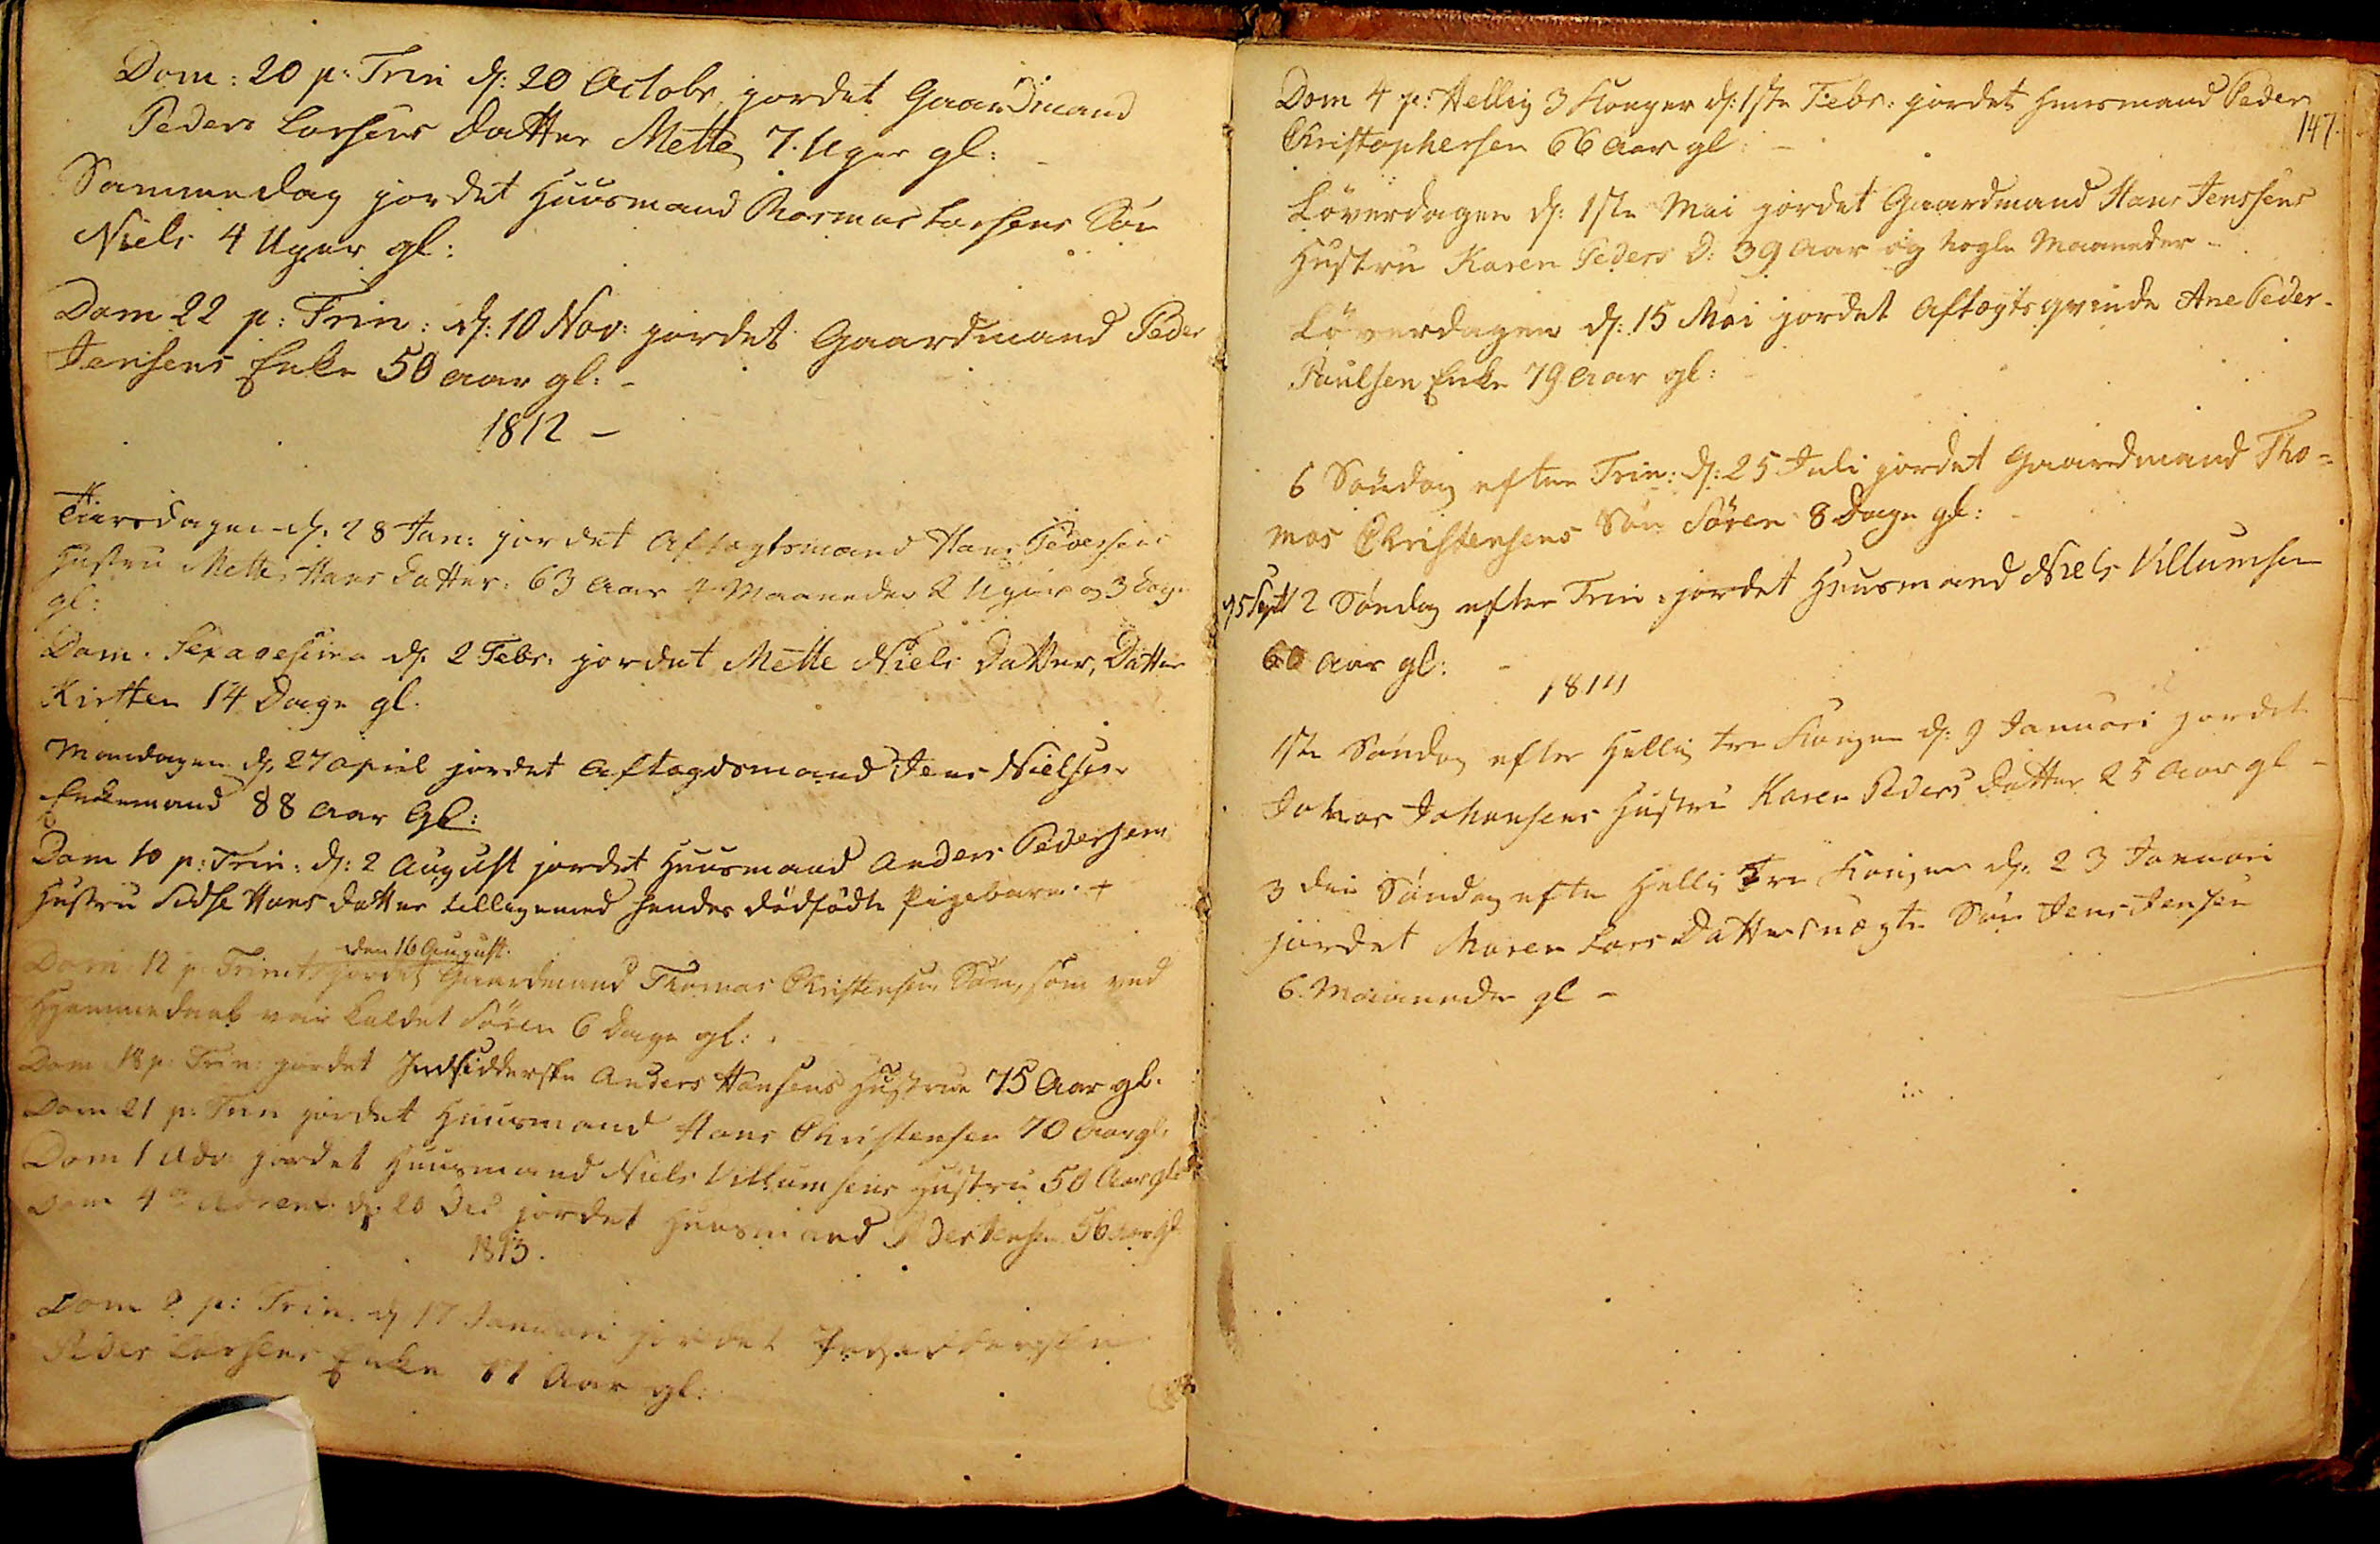Dom: 20 p: Trin d: 20 Octobr jordet Gaardmand
Peder Larsens Datter Mette 7 uger gl:
Sammedag jordet Huusmand Rasmus Larsens Søn
Niels 4 Uger gl:
Dom 22 p: Trin: d: 10 Nov: jordet Gaardmand Peder
Jensens Enke 50 Aar gl:
1812
Tirsdagen d: 28 Jan: jordet Aftægtsmand Hans Pedersens
Hustru Mette Hans Datter: 63 Aar 4 Maaneder 2 Uger og 3 Dage
gl:
Dom: Sexagesima d: 2 Febr: jordet Mette Niels Datter, Datter
Kirsten 14 Dage gl:
Mandagen d: 27 April jordet Aftægtsmand Jens Nielsen
Enkemand 88 Aar gl:
Dom 10 p: Trin: d: 2 August jordet Huusmand Anders Pedersens
Hustru Sidse Hans Datter tilligemed hendes dødfødte Pigebarn. +
den 16 August:
Dom: 12 p: Trinit jordet Gaardmand Thomas Christensens Søn, som ved
Hjemmedaab var kaldet Søren 6 Dage gl:
Dom: 18 p: Trin: jordet Indsiddererske Anders Hansens Hustrue 75 Aar gl:
Dom: 21 p: Trin jordet Huusmand Hans Christensen 70 Aar gl:
Dom: 1 Adv: jordet Huusmand Niels Villumsens Hustru 50 Aar gl:
Dom 4de Advent: d: 20 Dec: jordet Huusmand Peder Jensen. 56 Aar gl:
1813
Dom 2 p: Trin: d: 17 Januari jordet Indsidderske
Peder Larsens Enke 77 Aar gl:
147
Dom 4 p: Hellig 3 Konger d: 1ste Febr: jordet Huusmand Peder
Christophersen 66 Aar gl:
Løverdagen d: 1ste Mai jordet Gaardmand Hans Jensens
Hustru Karen Peders D: 39 Aar og nogle Maaneder
Løverdagen d: 15 Mai jordet Aftægtsqvinde Ane Peder
Poulsen Enke 79 Aar gl:
6 Søndag efter Trin: d: 25 Juli jordet Gaardmand Tho¬
mas Christensens Søn Søren 8 Dage gl:
d 5 Sept 12 Søndag efter Trin: jordet Huusmand Niels Villumsen
60 Aar gl:
1814
1ste Søndag efter Hellig tre Konger d: 9 Januari jordet
Jonas Johansens Hustru Karen Peders Datter 25 Aar gl
3die Søndag efter Hellig tre Konger d: 23 Januari
jordet Maren Lars Datters uægte Søn Jens Jensen
6 Maaneder gl
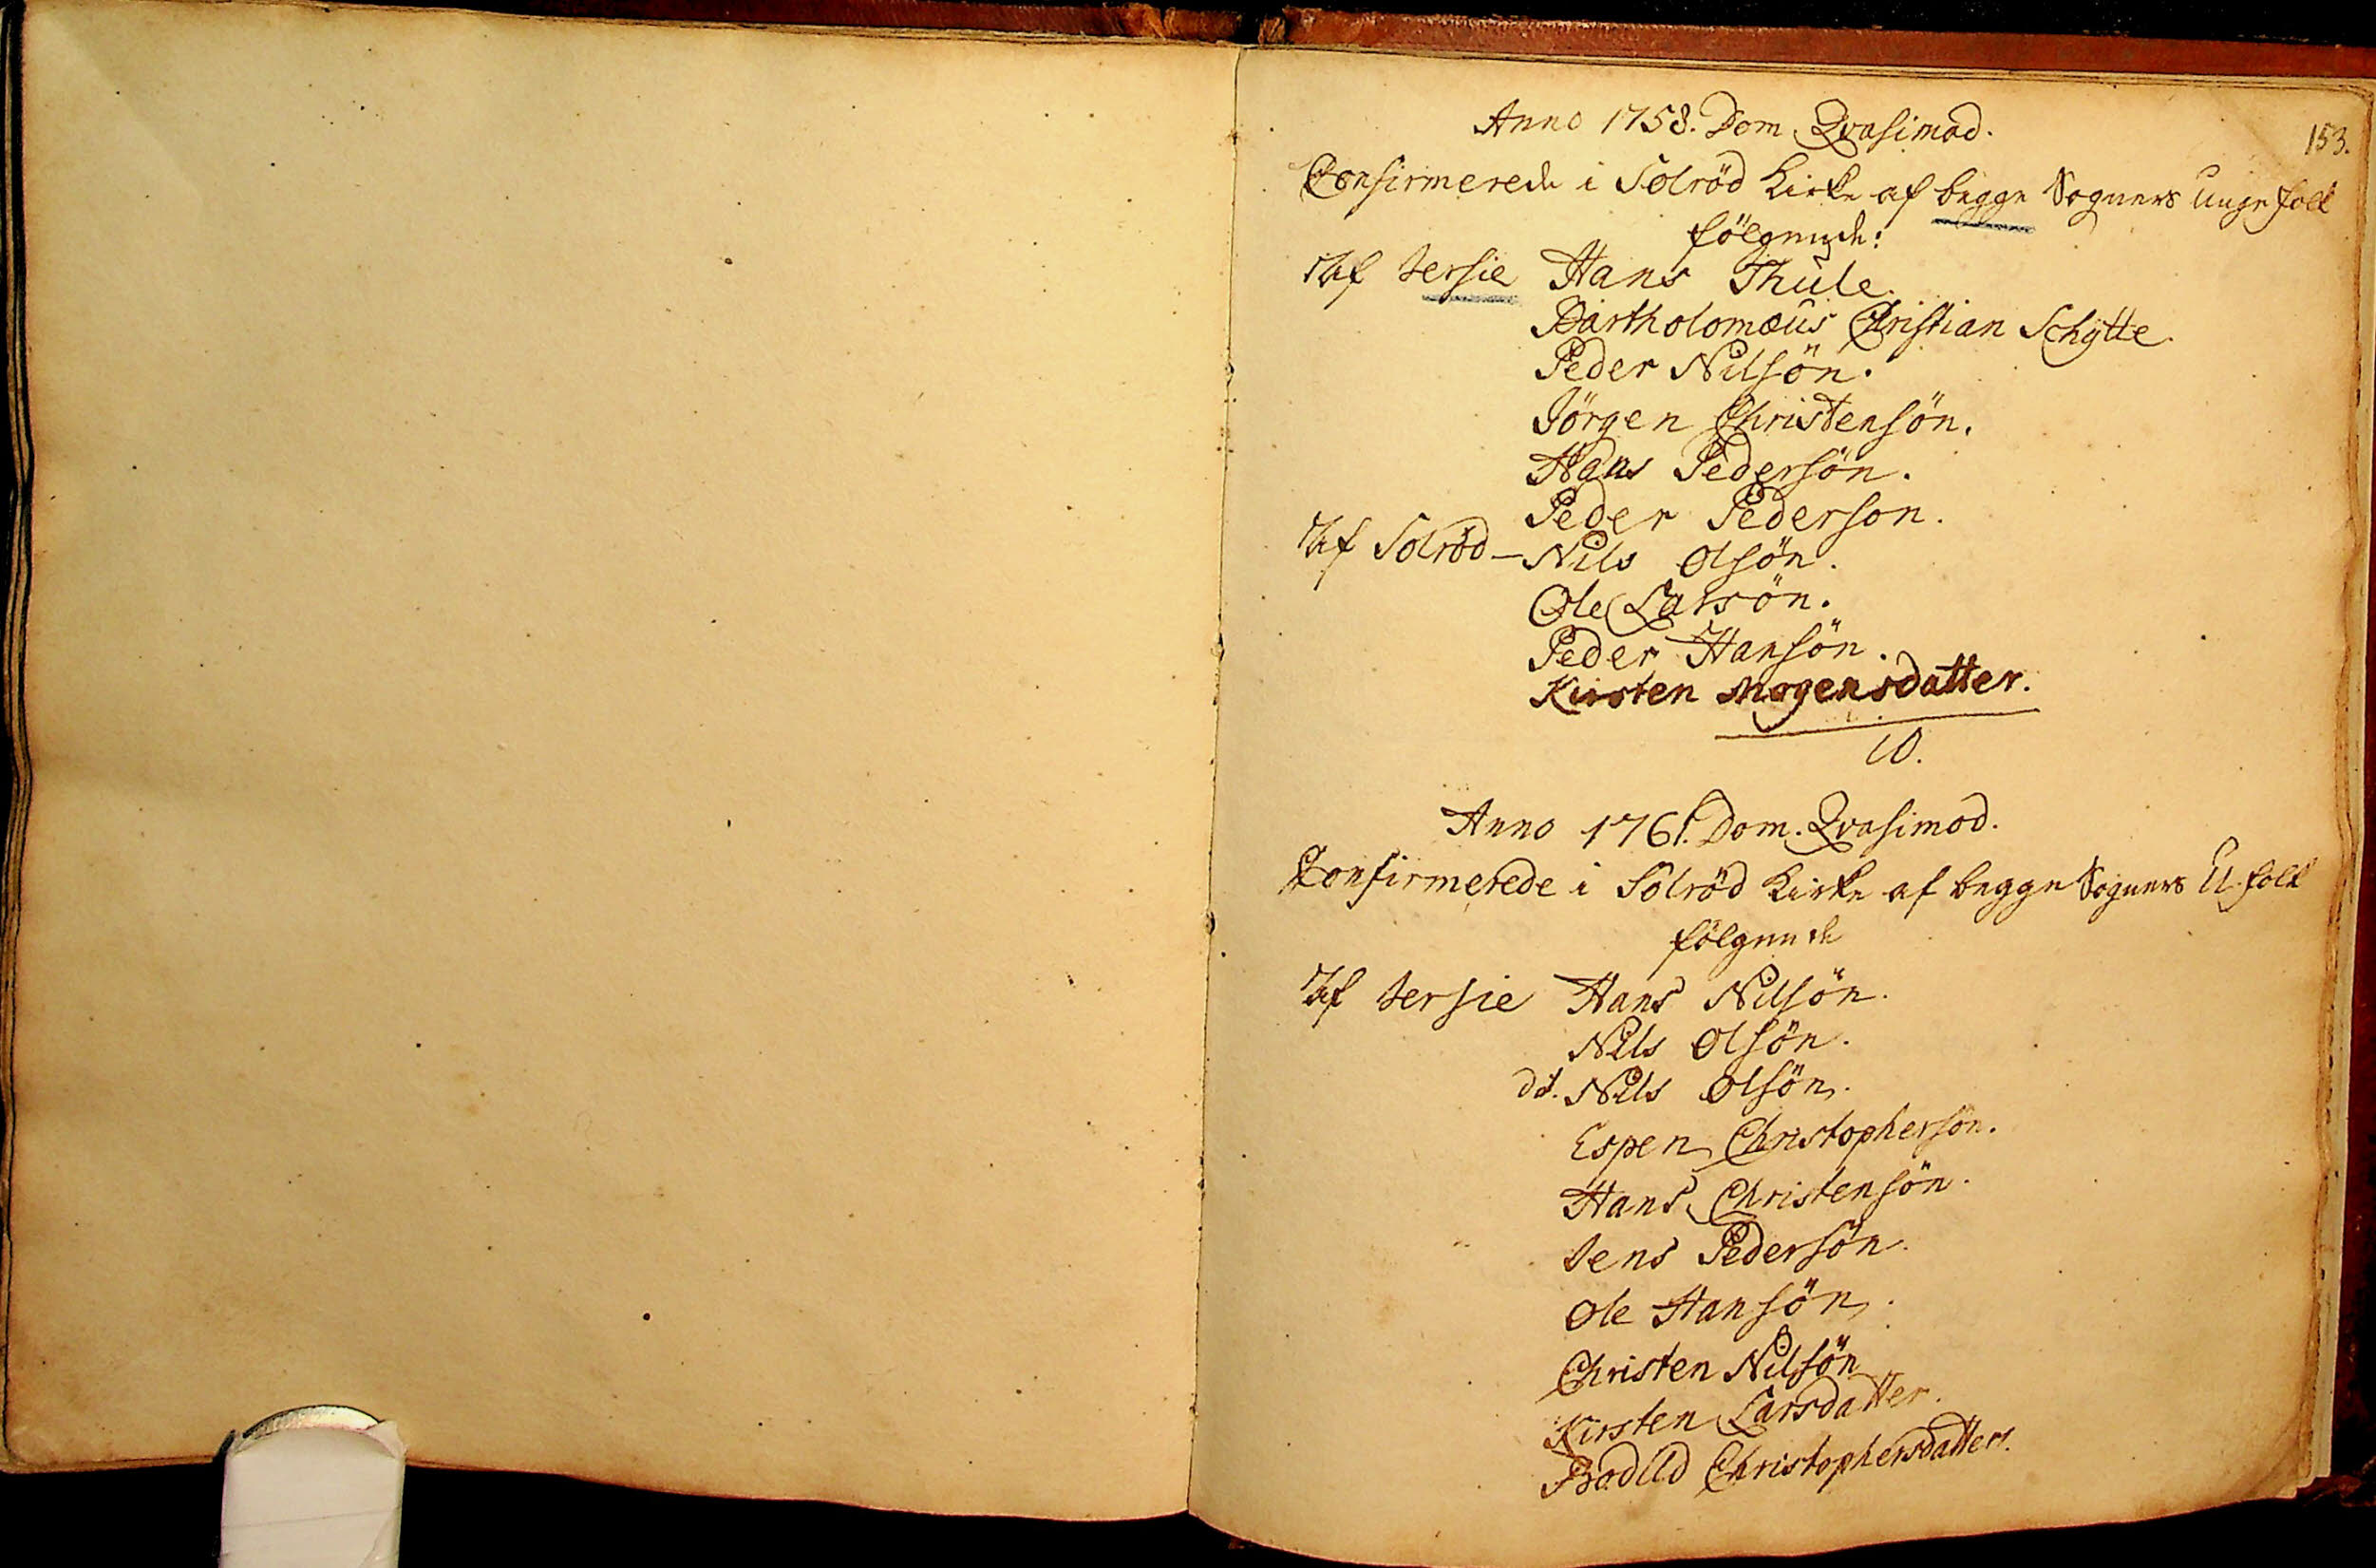153.
Anno 1758. Dom. Qvasimod.
Confirmerede i Solrød Kirke af begge Sogners Unge Folk
følgende:
Af Jersie Hans Thule.
Bartholomæus Christian Schytte.
Peder Nilsøn.
Jørgen Christensøn.
Hans Pedersøn.
Peder Pedersøn.
Af Solrød - Nils Olsøn.
Ole Larsøn.
Peder Hansøn.
Kirsten Mogensdatter.
10.
Anno 1761. Dom. Qvasimod.
Confirmerede i Solrød Kirke af begge Sognes U.Folk
følgende
Af Jersie Hans Nilsøn.
Nils Olsøn.
dt. Nils Olsøn.
Espen Christophersøn.
Hans Christensøn.
Jens Pedersøn.
Ole Hansøn.
Christen Nilsøn.
Kirsten Larsdatter.
Bodild Christophersdatter.